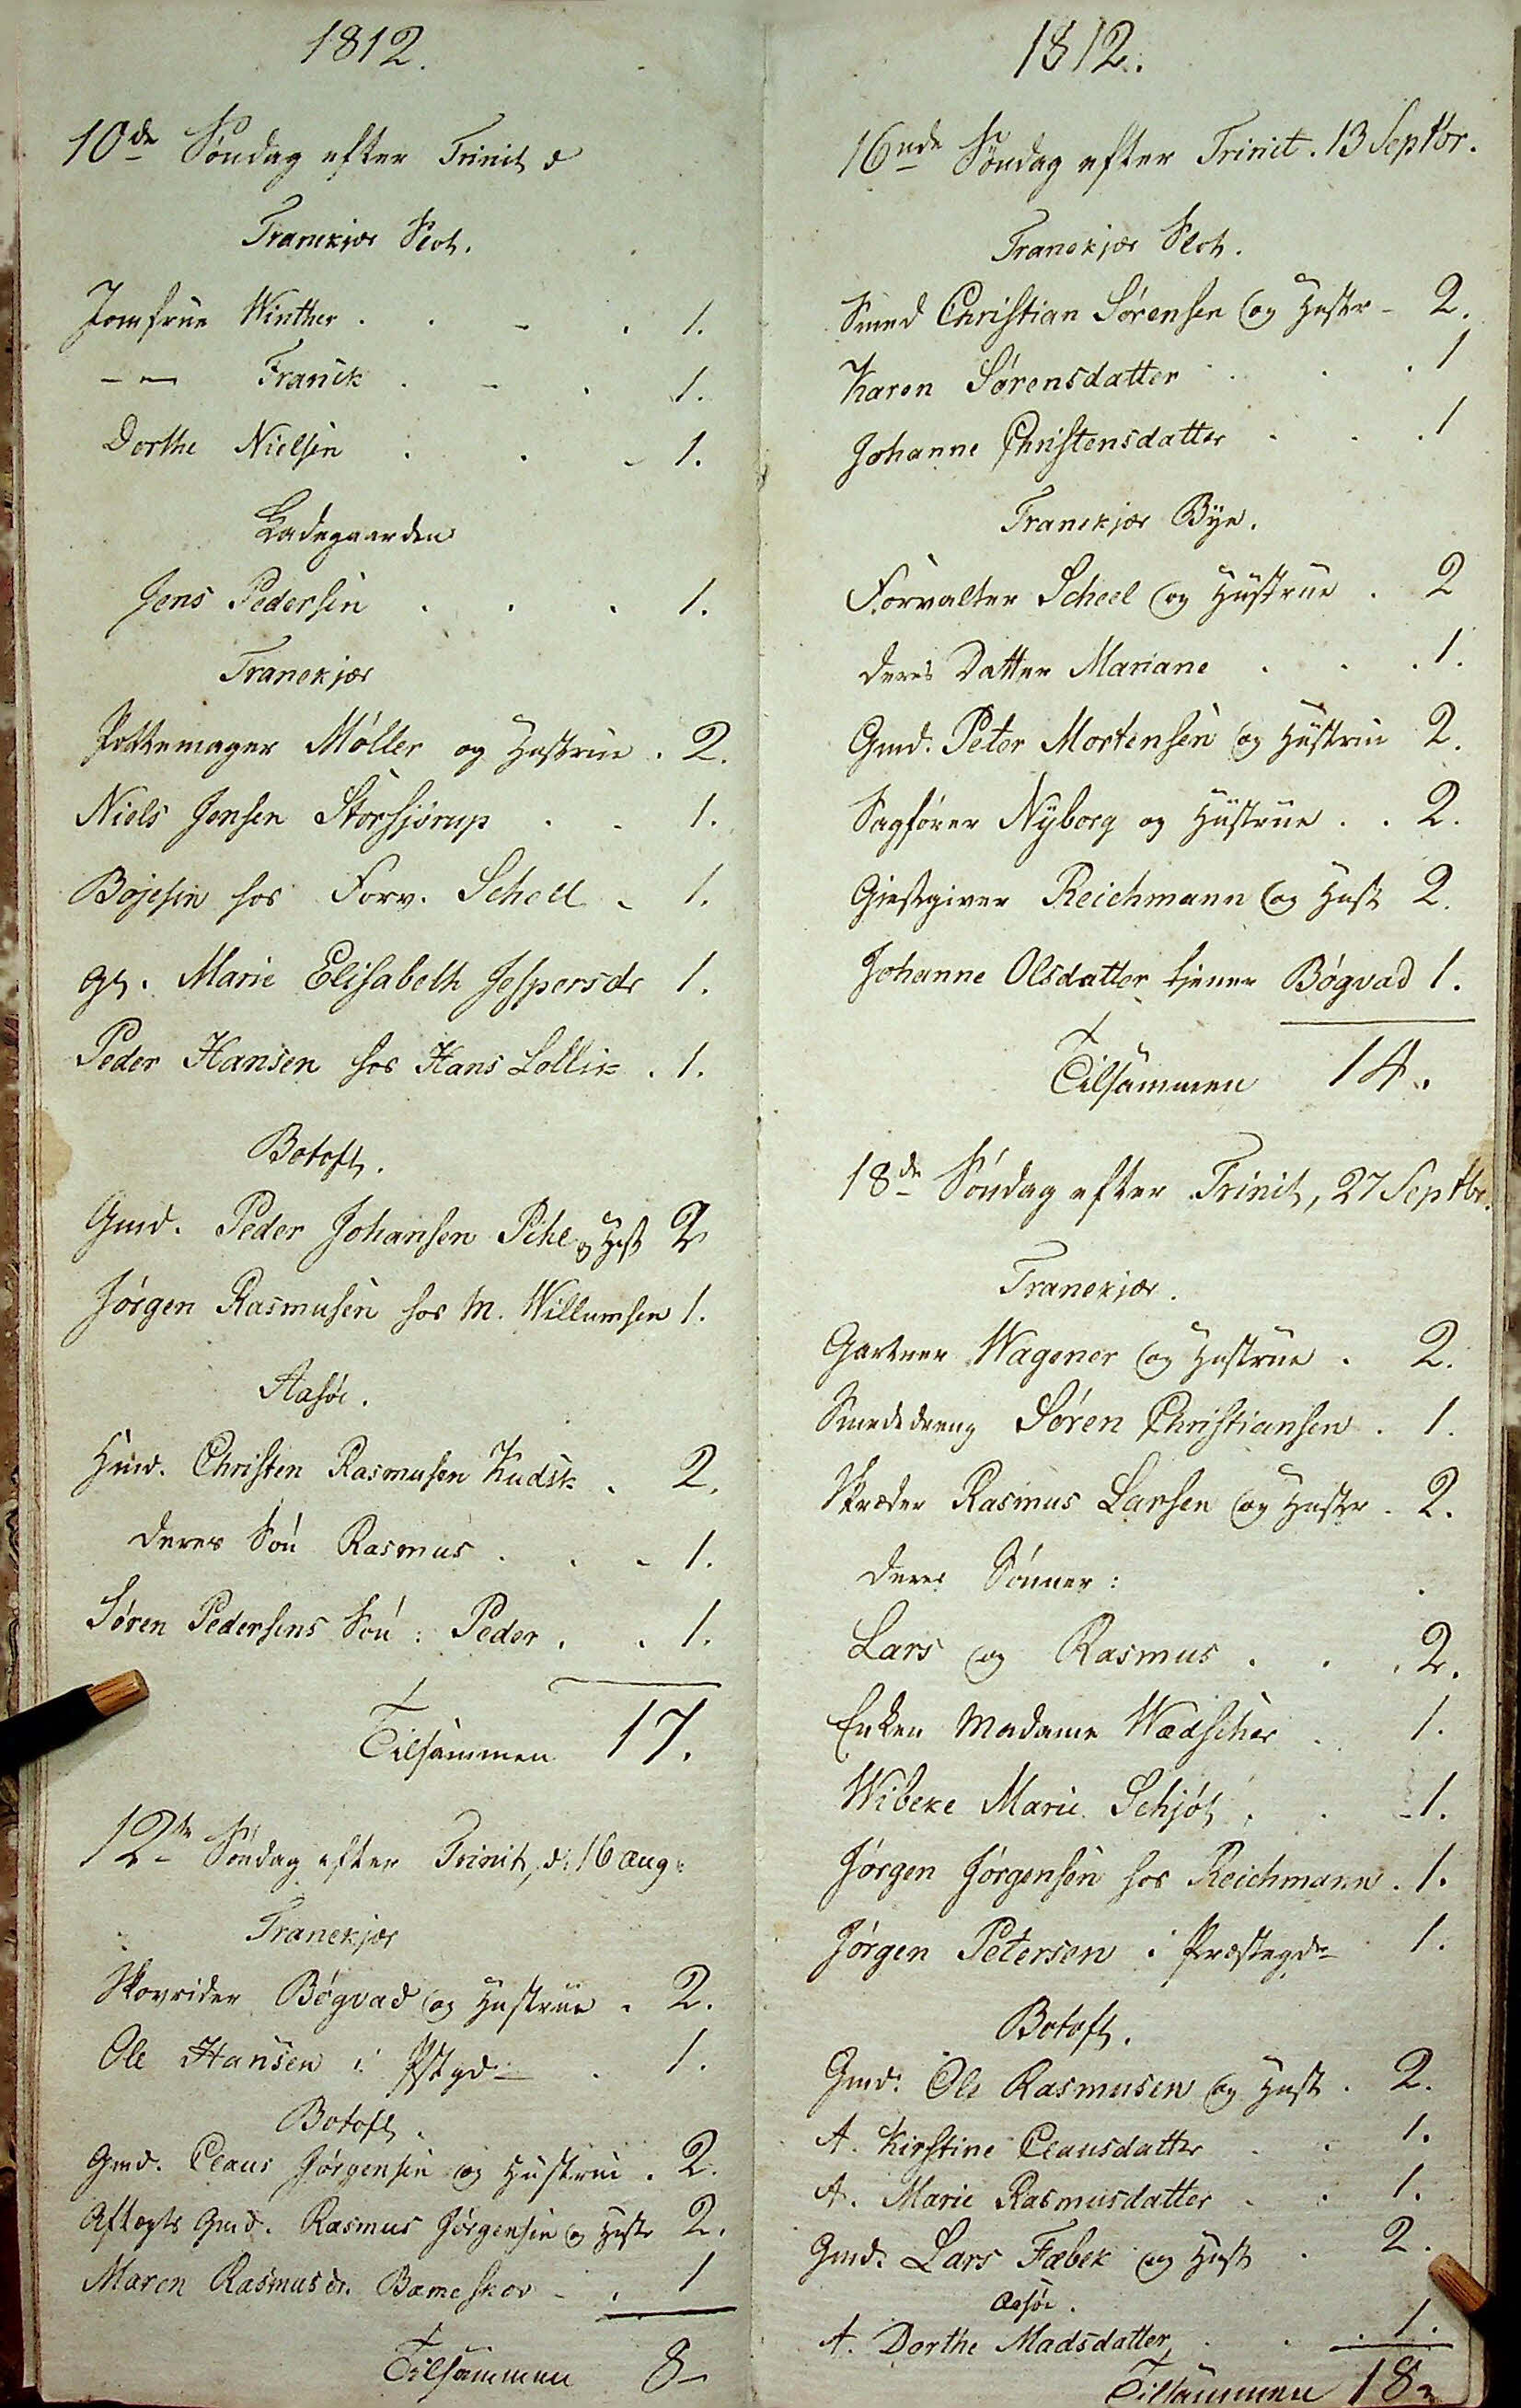1812.
10de Søndag efter Trinit d
Tranekjær Slot.
Jomfrue Winther . . . . 1.
-"- Franck . . . 1.
Dorthe Nielsen . . . 1.
Ladegaarden
Jens Pedersen . . . 1.
Tranekjær
Pottemager Møller og Hustrue . 2.
Niels Jensen Storsjørup . . 1.
Bojesen hos Forv. Schell . 1.
gl. Marie Elisabeth Jespersdr 1.
Peder Hansen hos Hans Lollik . 1.
Botoft.
Gmd. Peder Johansen Pihl og Hust 2
Jørgen Rasmusen hos M. Willumsen 1.
Aasøe
Hmd. Christen Rasmusens Kudsk . 2.
deres Søn Rasmus . . . 1.
Søren Pedersens Søn: Peder . . 1.
Tilsammen 17.
12te Søndag efter Trinit d: 16 Aug:
Tranekjær.
Skovrider Bøgvad og Hustrue . 2.
Ole Hansen i Pstgd - . 1.
Botoft.
Gmd. Claus Jørgensen og Hustrue . 2.
Aftægts Gmd. Rasmus Jørgensen og Hustr 2.
Maren Rasmusdr Bameskov . . 1
Tilsammen 8 -
1812.
16nde Søndag efter Trinit. 13 Septbr.
Tranekjær Slot.
Smed Christian Sørensen og Hustr - 2.
Karen Sørensdatter . . . 1
Johanne Christensdatter . . . 1
Tranekjær Bye.
Forvalter Scheel og Hustrue . 2
deres Datter Mariane . . . 1.
Gmd. Peter Mortensen og Hustrue 2.
Sagfører Nyborg og Hustrue . . 2.
Giestgiver Reichmann og Hust 2.
Johanne Olsdatter tjener Bøgvad 1.
Tilsammen 14.
18de Søndag efter Trinit, 27 Septbr.
Tranekjær.
Gartner Wagener og Hustrue . 2.
Smededreng Søren Christiansen . 1.
Skræder Rasmus Larsen og Hustr . 2.
deres Sønner:
Lars og Rasmus . . . 2.
Enken Madame Wædscher . 1.
Wibeke Marie Schjøt . . 1.
Jørgen Jørgensen hos Reichmann . 1.
Jørgen Petersen i Præstegdr . 1.
Botoft.
Gmd. Ole Rasmusen og Hust . 2.
A. Kirstine Clausdatter . . 1.
A. Marie Rasmusdatter . . 1.
Gmd Lars Fæbek og Hust . 2.
Aasøe
A. Dorthe Madsdatter . . . 1.
Tilsammen 18.
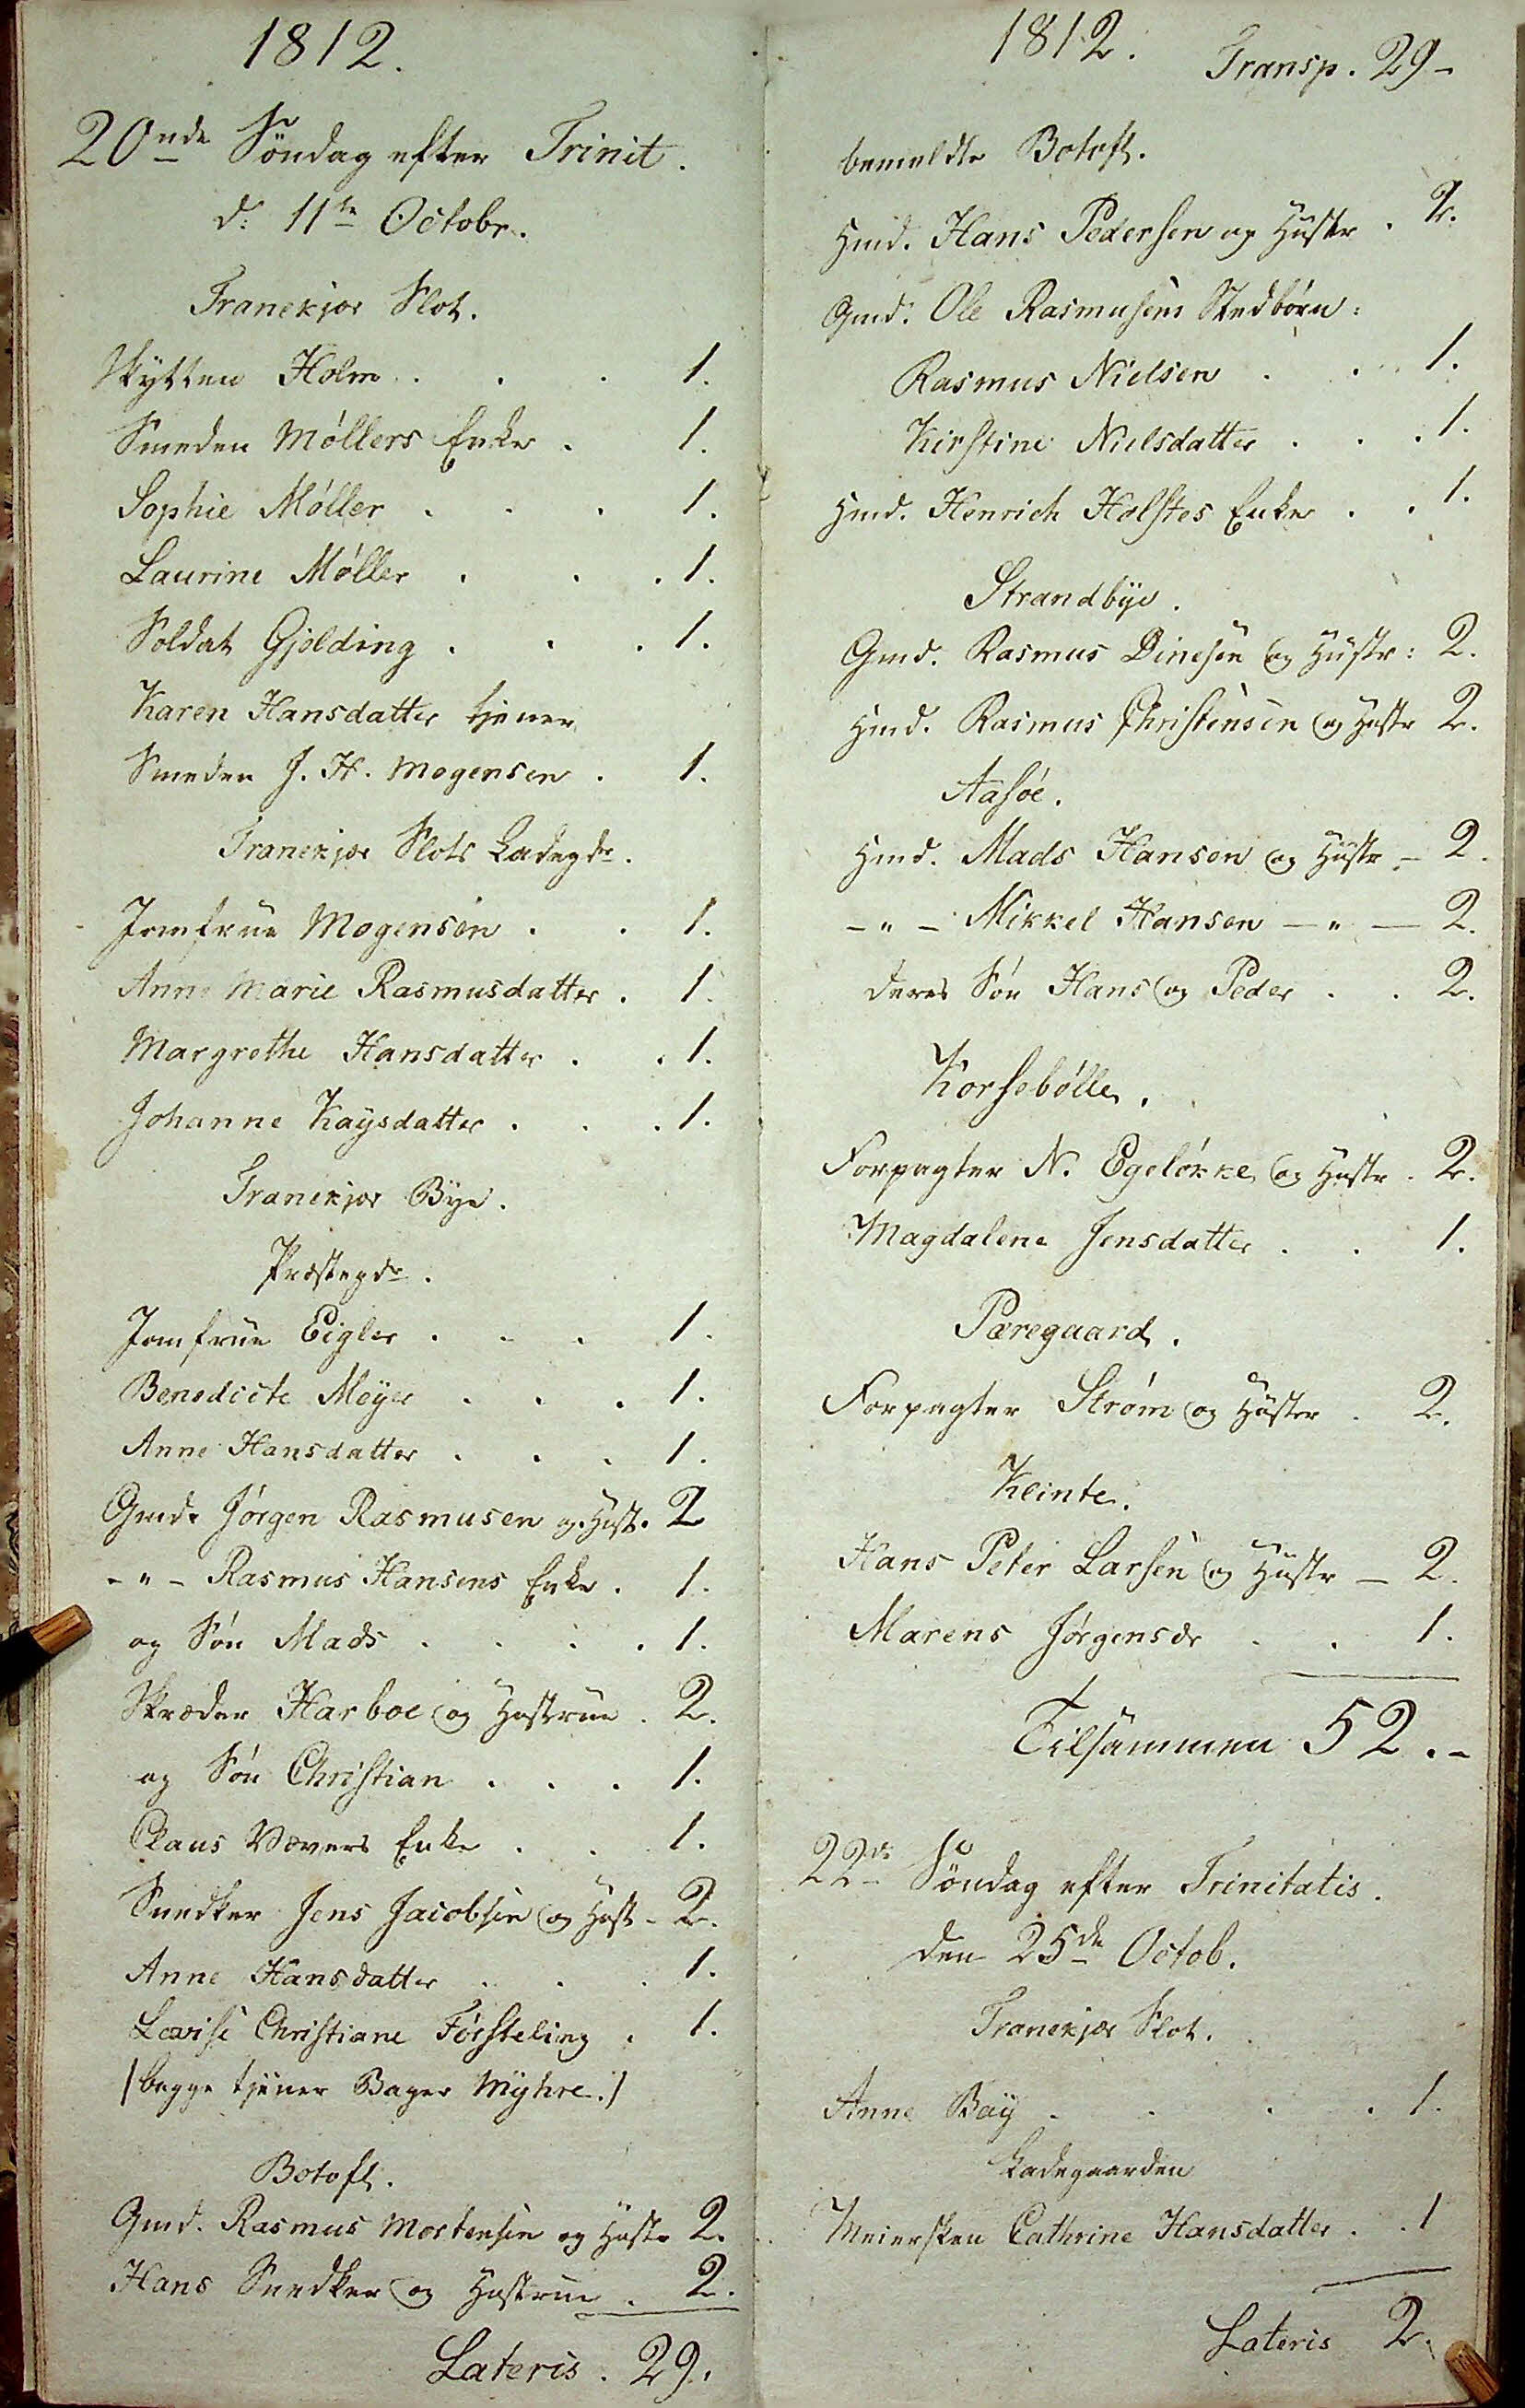1812
20nde Søndag efter Trinit.
d: 11te Octobr.
Tranekjær Slot
Skytten Holm . . . 1.
Smeden Møllers Enke . 1.
Sophie Møller . . . 1.
Laurine Møller . . . 1.
Soldat Gjølding . . . 1.
Karen Hansdatter tjener
Smeden J. H. Mogensen . 1.
Tranekjær Slots Ladegdr.
Jomfrue Mogensen . . 1.
Anne Marie Rasmusdatter . 1.
Margrethe Hansdatter . . 1.
Johanne Kaysdatter . . . 1.
Tranekjær Bye.
Præstegdr.
Jomfrue Eigler . . . 1.
Benedicte Meyer . . . 1.
Anne Hansdatter . . . 1.
Gmd. Jørgen Rasmusen og Hust . 2
-"- Rasmus Hansens Enke . 1.
og Søn Mads . . . . 1.
Skræder Harboe og Hustrue . 2.
og Søn Christian . . . 1.
Claus Vævers Enke . . 1.
Snedker Jens Jacobsen og Hust . 2.
Anne Hansdatter . . . 1.
Lovise Christian Førsteling . 1.
|begge tjener Bager Myhre. |
Botoft.
Gmd. Rasmus Mortensen og Hustr 2.
Hans Snedker og Hustrue . 2
Lateris. 29.
1812.
Transp. 29 -
bemeldte Botoft.
Hmd. Hans Pedersen og Hustr . 2.
Gmd. Ole Rasmusens Stedbørn:
Rasmus Nielsen . . . 1.
Kirstine Nielsdatter . . . 1.
Hmd. Henrich Holstes Enke . . 1.
Strandbye
Gmd. Rasmus Dinesen og Hustr : 2.
Hmd. Rasmus Christensen og Hustr 2.
Aasøe.
Hmd. Mads Hansen og Hustr - 2
-"- Mikkel Hansen - " - 2.
deres Søn Hans og Peder . . 2.
Korsebølle.
Forpagter N. Egeløkke og Hustr . 2.
Magdalene Jensdatter . . 1.
Pæregaard.
Forpagter Strøm og Hustr . 2.
Klinte.
Hans Peder Larsen og Hustr - 2.
Marens Jørgensdr . . 1.
Tilsammen 52.
22de Søndag efter Trinitatis.
den 25de Octob.
Tranekjær Slot
Anne Bay . . . . 1.
Ladegaarden
Meiersken Cathrine Hansdatter . . 1
Lateris 2.